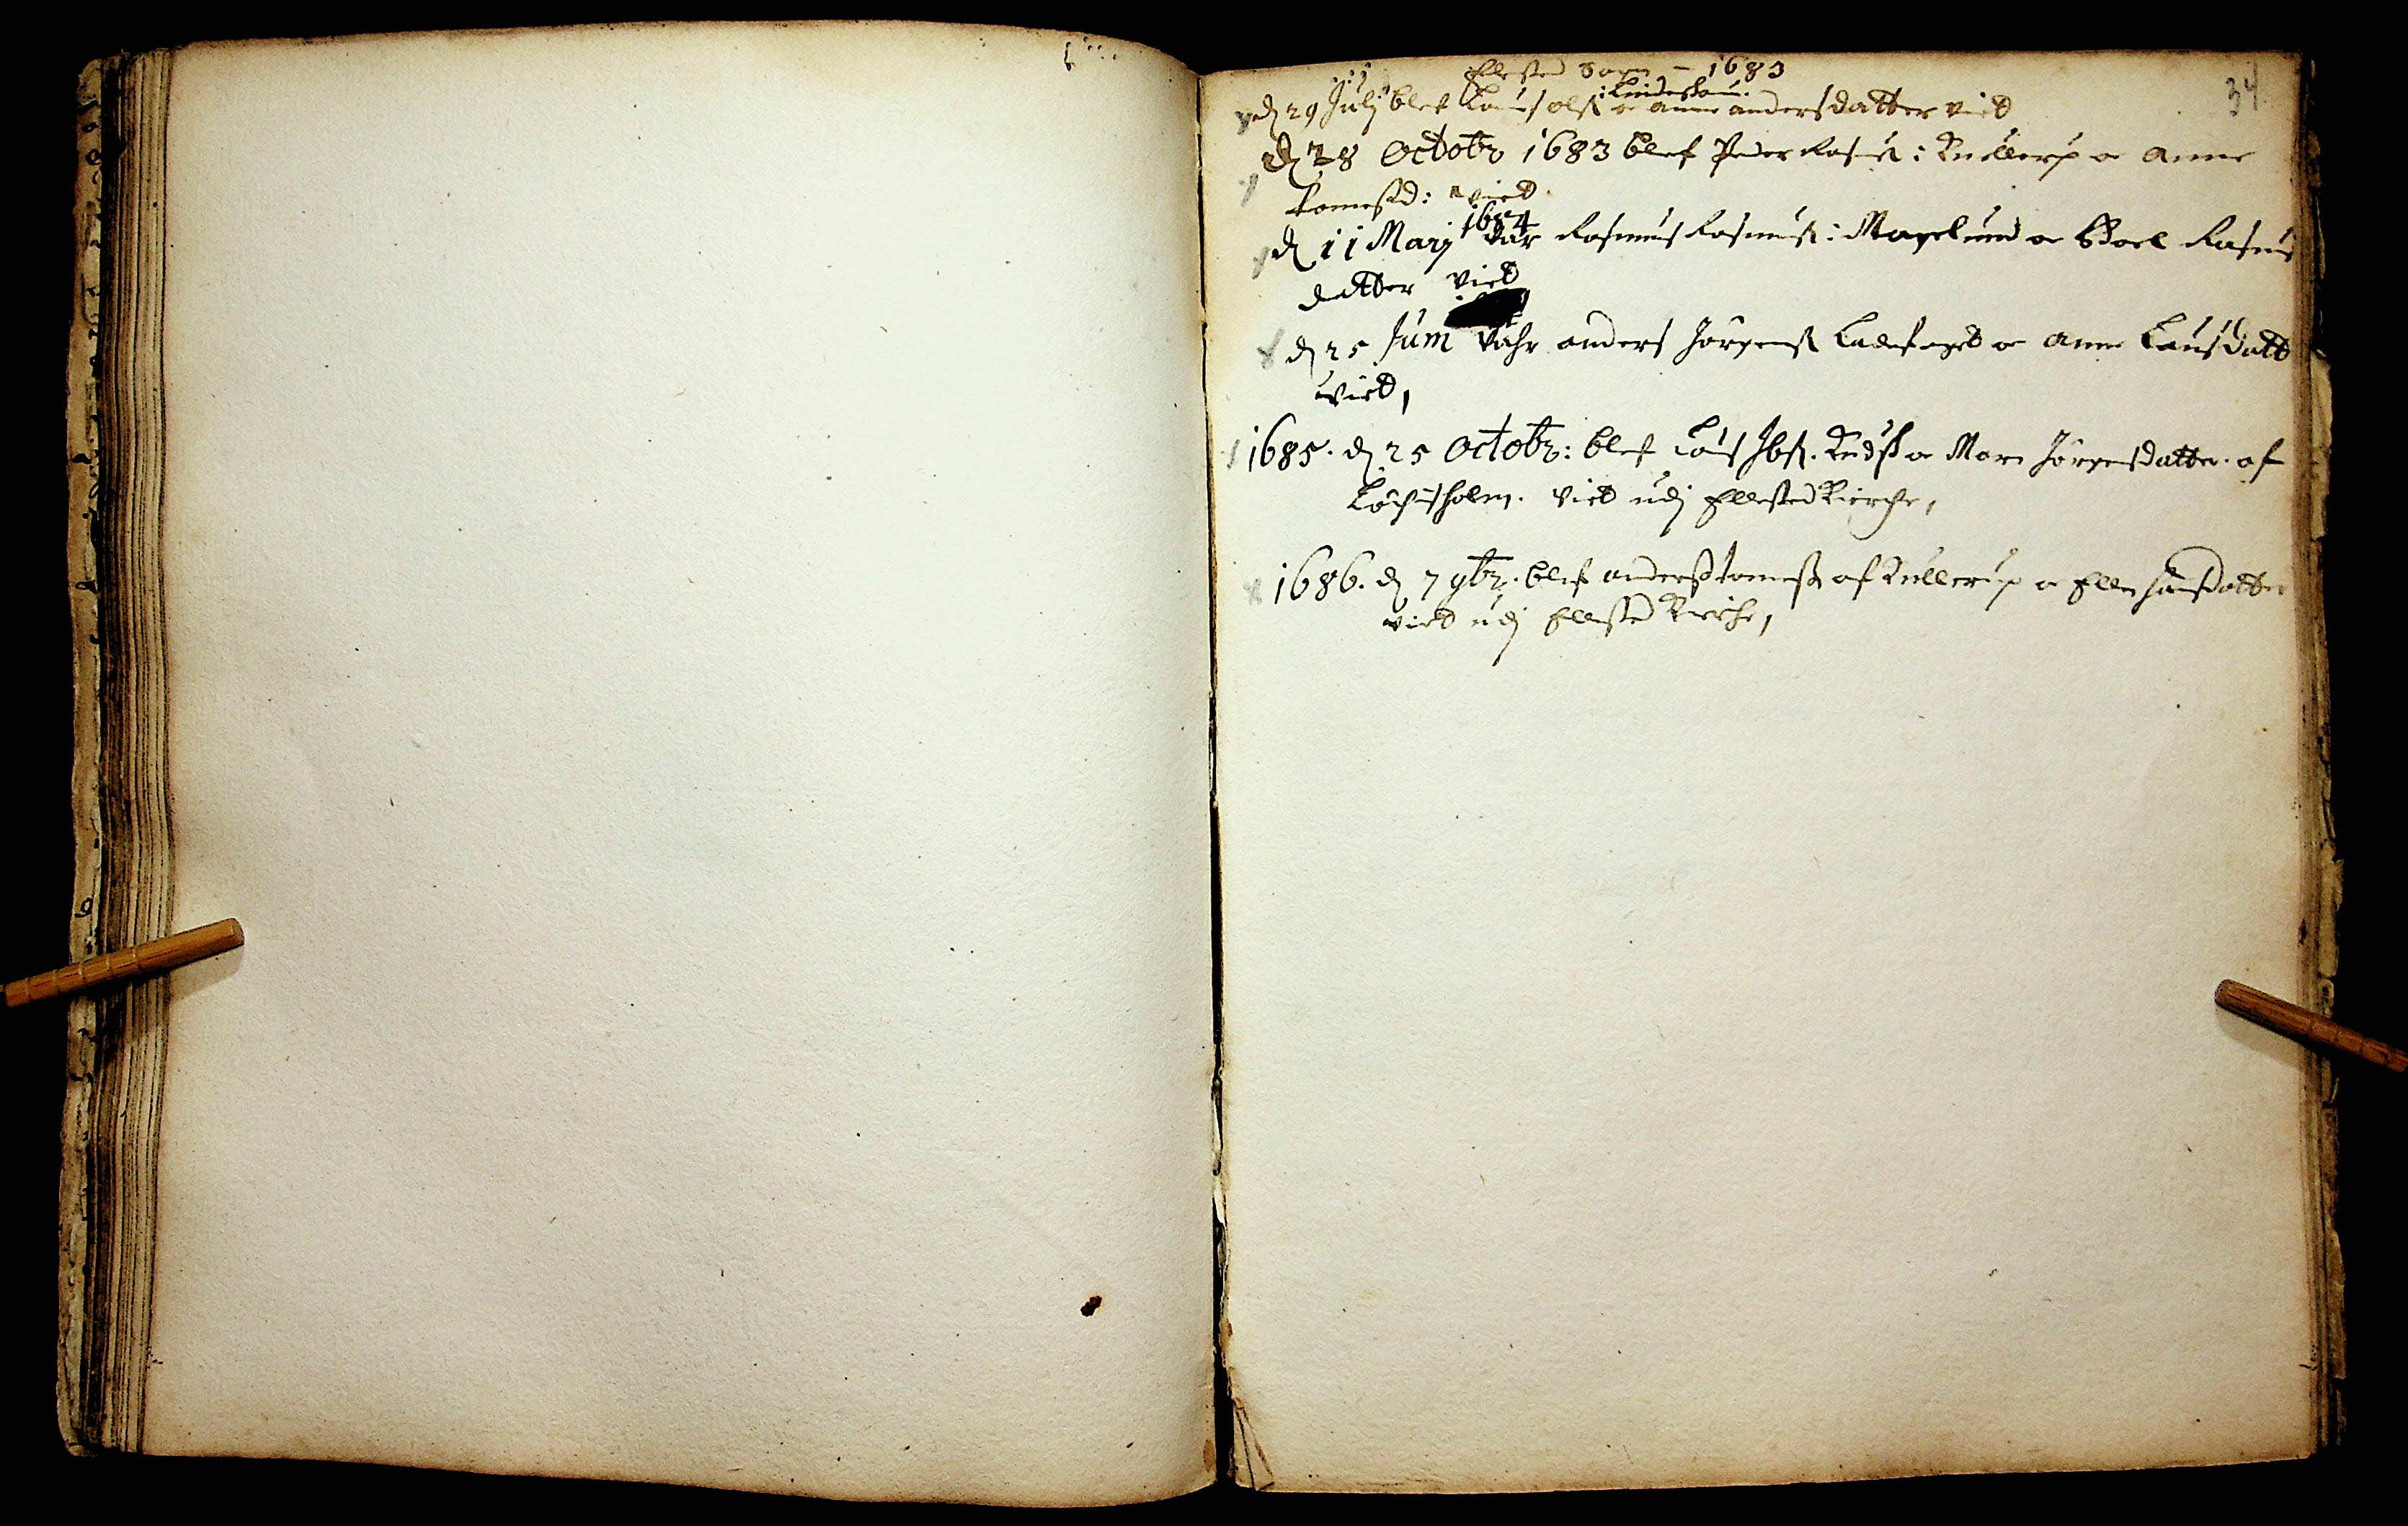34.
Elested Sogn - 1683
i Lindeskou.
d 29 Julij blef Laus Ols oc Anne Andersdatter viet
D 28 Octobr 1683 blef Peder Rasmus i Kullerp oc Anne
Tomesd: viet
1684
d 11 Marij: var Rasmus Rasmus: Magelund oc Boel Rasmus
datter viet
d 25 Juni Vahr anders Jørgens Ladefoget oc Anne Lausdatter
viet
1685. d 25 Octobr: blef Laus Ibs. kudsk oc Maren Jørgensdatter af
Løchisholm. viet udi Ellested Kirche,
1686. d 7 9br. blef Anderss tomess af Kullerup oc Ellen Hansdatter.
viet udi Ellested Kirche,
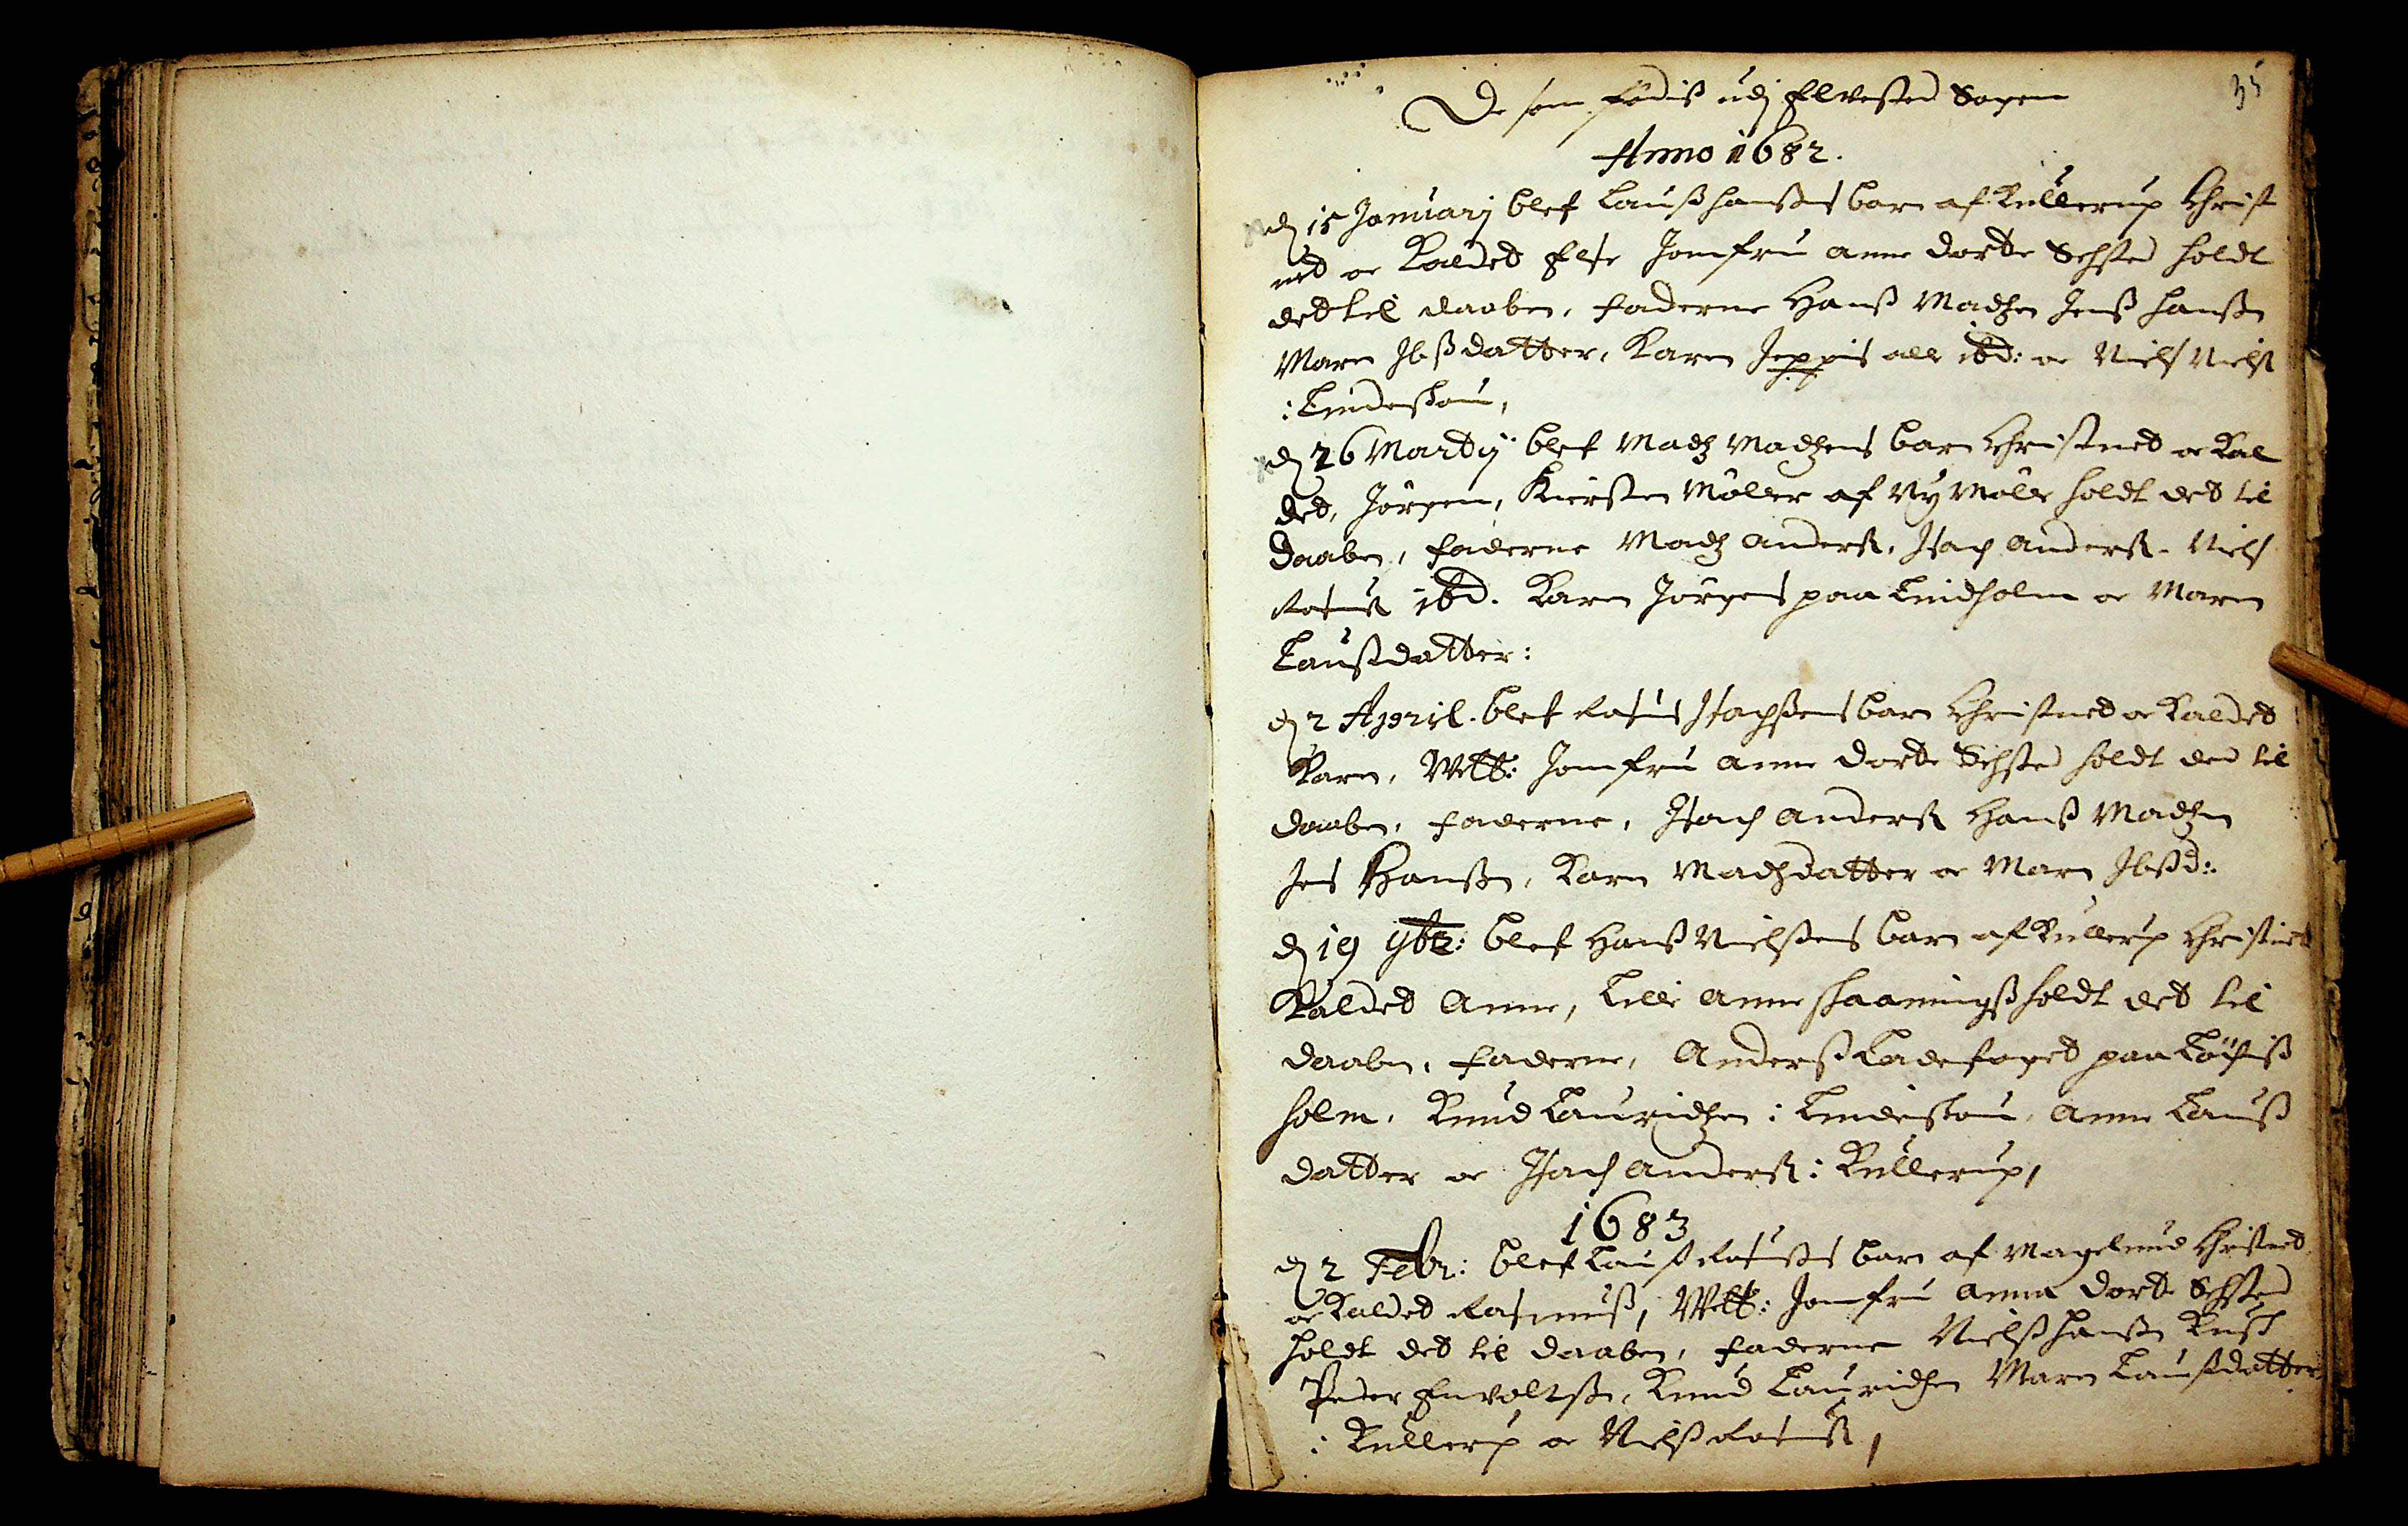35
De som fødis udi Ellested Sogen
Anno 1682
d 15 Januarij bleff Laus hansens barn af Kullerup Christ
net oc kaldet Else Jomfru Anne Dorte Sehsted holdt
det tel daaben, Faderne Hanss Madtzen Jenss Hanssen
Maren Jbssdatter, Karen Jeppis alle ibd: oc Niels Nielss
i Lindeskou,
d 26 Martij blef Madtz Madtzens barn Christned oc Kal
det, Jørgen Kiersten Møller af Ny Mølle holdt det til
daaben, Faderne Madtz Anders, Isach Anders Niels
Rasmus ibd. Karen Jørgens paa Lindholm oc Maren
Laussdatter:
d 2 April blef Rasmus Jsachsens barn Christned oc kaldet
Karen, Welb: Jomfru Anne Dorte Sehsted holdt det til
daaben. Faderne, Isach Anders Hans Madtzen
Jens Hansssn, Karen Madsdatter oc Maren Ibssd:
d 19 9br: blef Hanss Nielsens barn af Kullerup Christnet
kaldet Anne, Lille Anne skaaningss sholdt det til
daaben. Faderne, Anderss Ladefoget paa Løchiss
holm. Knud Lauridtzen i Lindeskou, Anne Lauss
datter oc Isach Anderss i Kullerup,
1683
d 2 Febr: blef Lauss Rasmussens barn af Magelund Christenet
oc kaldet Rasmuss, Welb: Jomfru Anne Dorte Sehfsted
holdt det til daaben, Faderne Niels Hansen Kusk
Peder Envoltss, Knud Lauridtzen, Maren Lausdatter
i Kullerup oc Nielss Rasmuss,